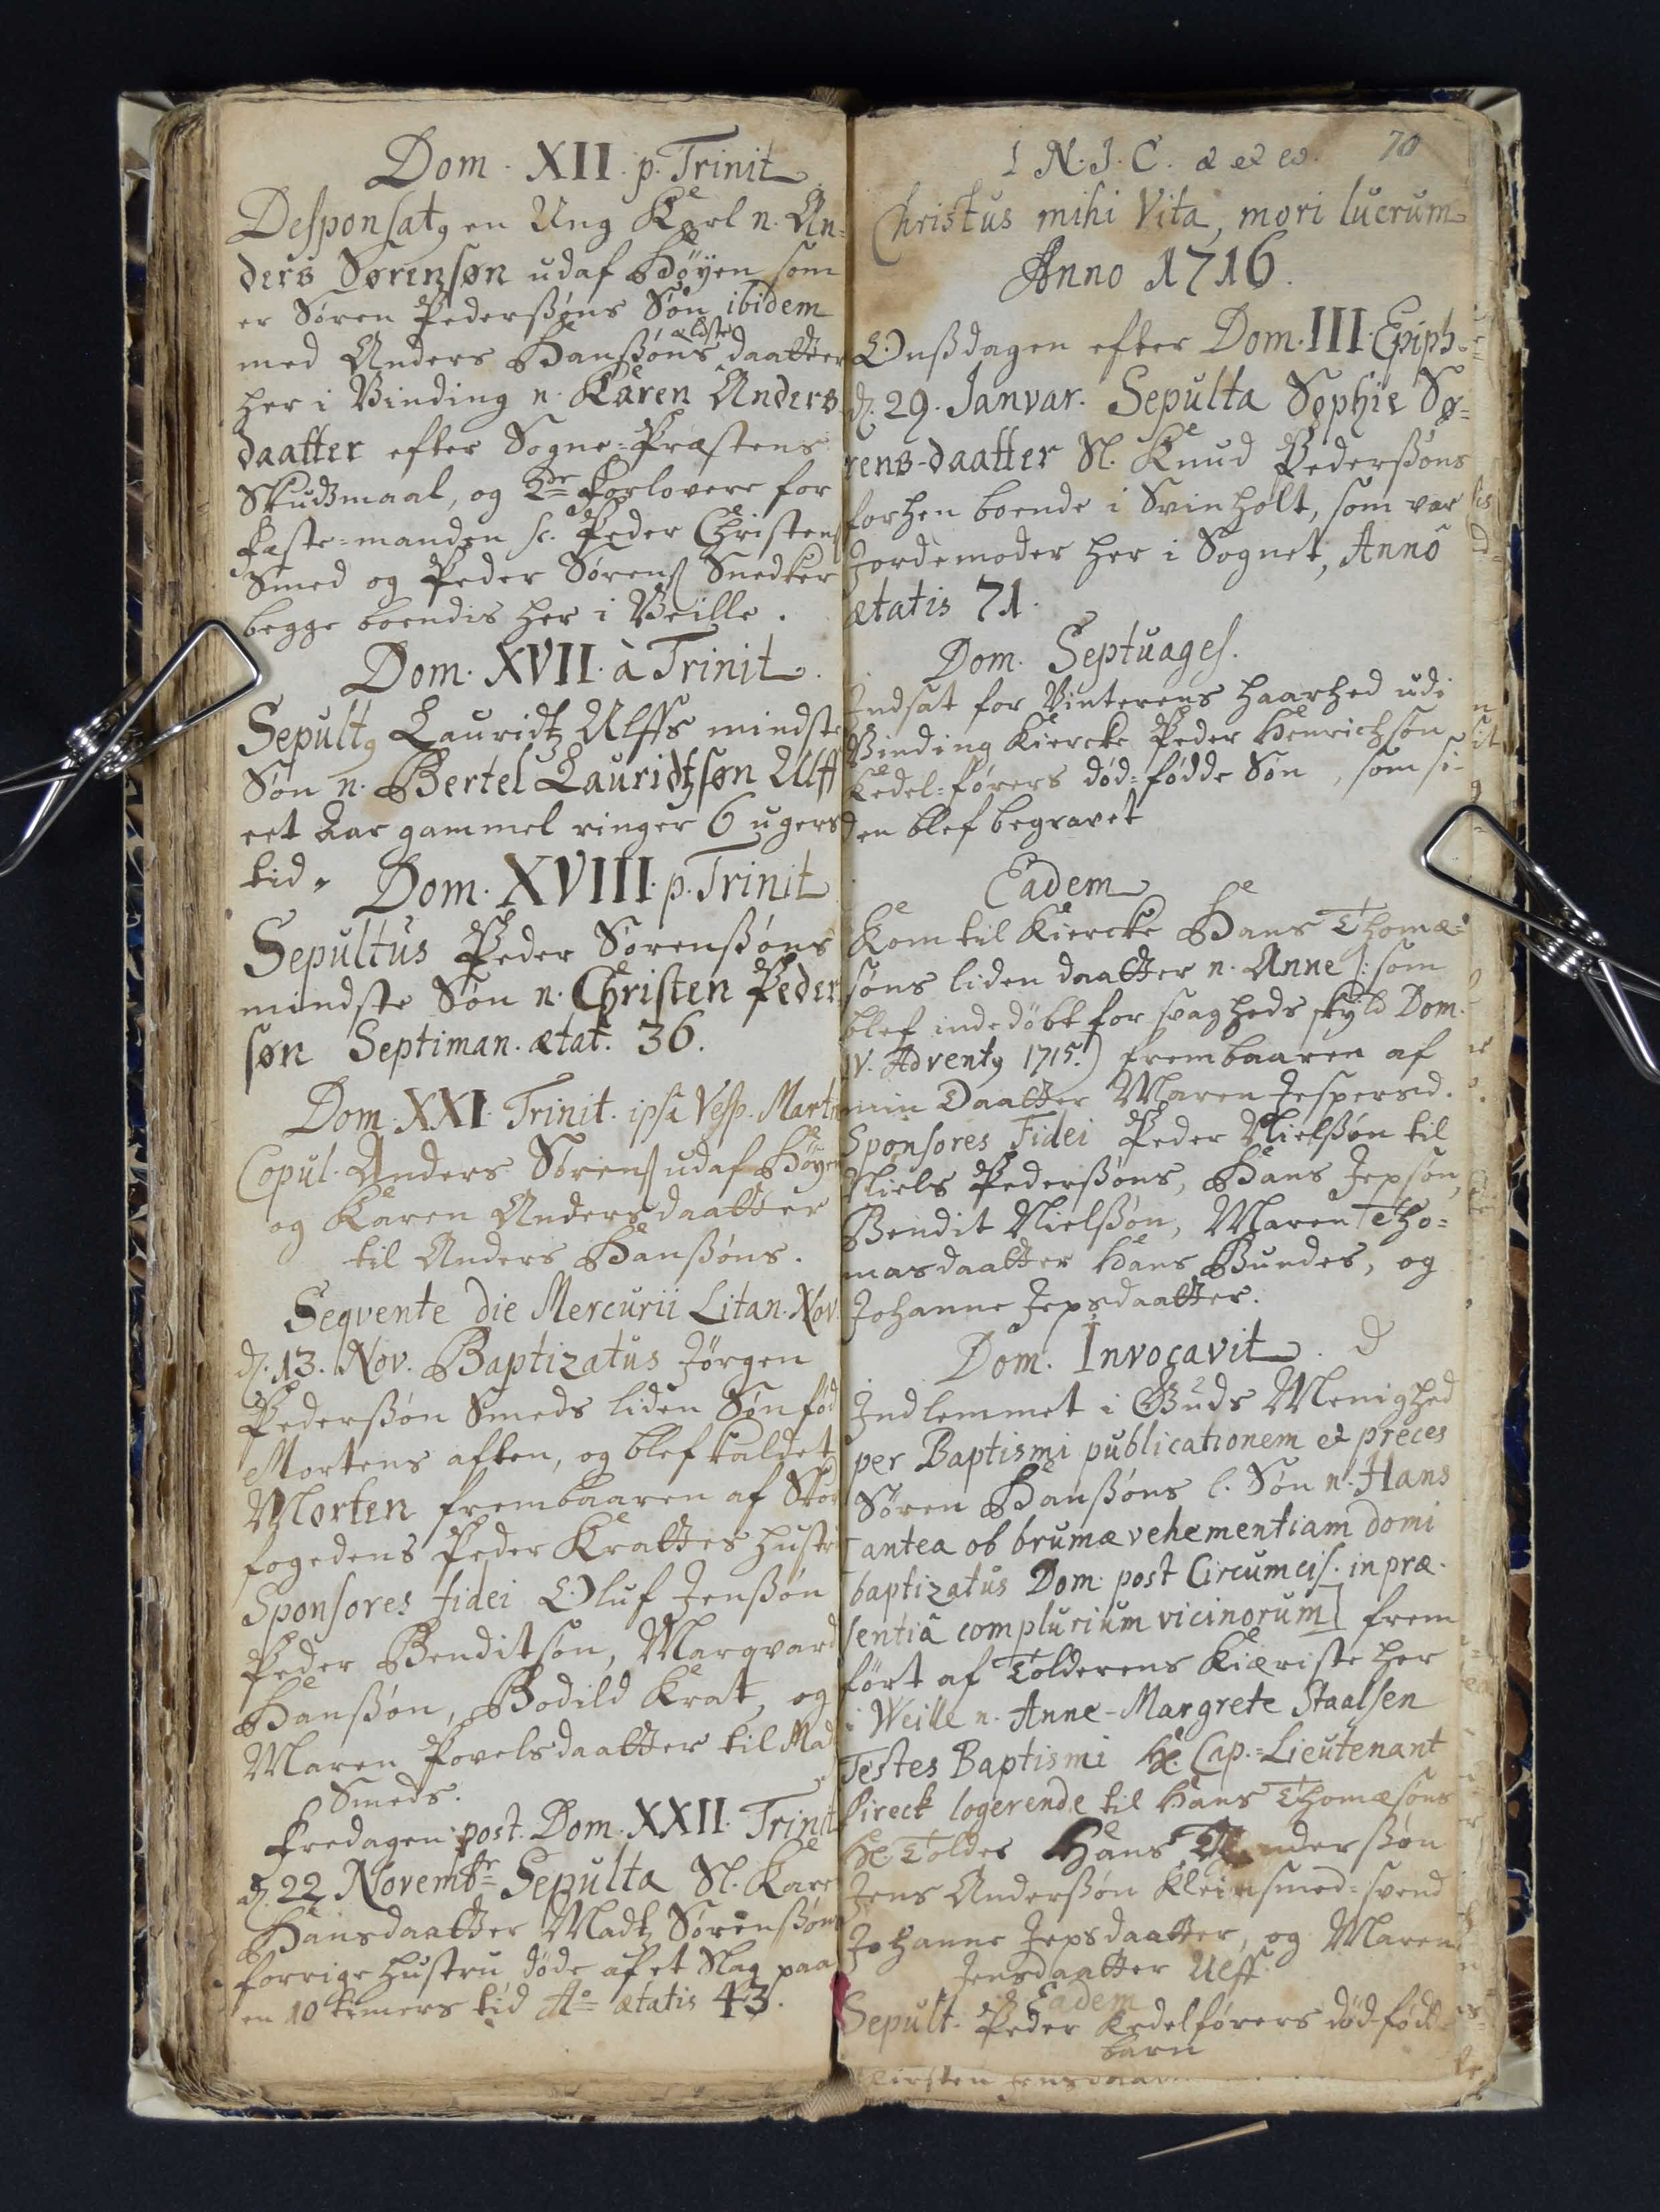Dom. XII. p. Trinit
Desponsatq en Ung Karl n. An
ders Sørensøn udaf Høyen som
er Søren Pederssøns Søn ibidem
ældste
med Anders Hanssøns daatter
her i Vinding n. Karen Anders
datter efter Sogne=Præstens 
Skudsmaal, og 2de Forlovere for
Fæste=manden sc. Peder Christens
Smed og Peder Sørens Snedker
begge boendis her i Veille.
Dom. XVII. a Trinit
Sepultq Lauridtz Ulffs mindste
Søn n. Bertel Lauridtzsøn Ulff
eet Aar gammel ringer 6 ugers
tid.
Dom. XVIII. p. Trinit
Sepultus Peder Sørenssøns
mindste Søn n. Christen Peder¬
søn Septiman. ætat. 36.
Dom. XXI Trinit. ipsa Vesap Martii
Copul. Anders Sørens udaf Høyen
og Karen Andersdaatter
til Anders Hanssøns.
Seqvente die Mercurii Litan. Nov.
d. 13. Nov. Baptizatus Jørgen
Pederssøn Smeds liden Søn fød
Mortens aften, og blef kaldet
Morten, frembaaren af Skov
fogedens Peder Krattis hustru 
Sponsores fidei Oluf Jenssøn
Peder Benditsøn, Margvard
Hanssøn, Bodild Krat, og
Maren Povelsdaatter til Mads 
Smeds.
Fredagen post Dom. XXII. Trinit
d. 22 Novembr Sepulta Sl. Karn 
Hansdaatter Madtz Sørenssøns
forrige hustru, døde af et Slag paa 
en 10 timers tid Ao ætatis 43.
70
I N. J. C. a et ##
Christus mihi Vita, mori lucrum
Anno 1716
Onssdagen efter Dom. III Epiph.
d. 29. Janvar. Sepulta Sophie Sø¬
rens-daatter Sl. Knud Pederssøns
forhen boende i Svinholt, som var 
Jordemoder her i Sognet, Anno 
ætatis 71
Dom. Septuages.
Indsat for Vinterens haarhed udi
Vinding Kiercke Peder Henrichsøn 
Kedel=førers død=fødde Som, som si¬
den blef begravet
Eadem
Kom til Kiercke Hans Thomæ¬
søns liden daatter n. Anne /: som
blef indedøbt for svagheds skyld Dom.
IV. Adventq 1715.) frembaaren af
min Daatter Maren Jespersd.
Sponsores Fidei Peder Nielsøn til
Niels Pederssøns, Hans Jepsøn,
Bendit Nielssøn, Maren Tho¬
masdaatter Hans Bundes, og
Johanne Jepsdaatter.
Dom. Invocavit
Indlemmet i Guds Menighed
per Baptismi publicationem et preces
Søren Hanssøns l. Søn n. Hans
[antea ob brumæ vehementiam domi
baptizatus Dom. post Circumcis. in præ¬
sentia complurium vicinorum] frem
ført af Tolderens Kiæriste her
i Weille n. Anne-Margrete Staalsen
Testes Baptismi Hl Cap.=Lieutenant
##ireck logerende til Hans Thomæsøns
Hl. Toldes Hans ##nderssøn
Jens Anderssøn Kleinsmed=svend
Johanne Jepsdaatter, og Maren
Jensdaatter Ulff.
Eadem
Sepult. Peder Kedelførers død-fødte 
barn
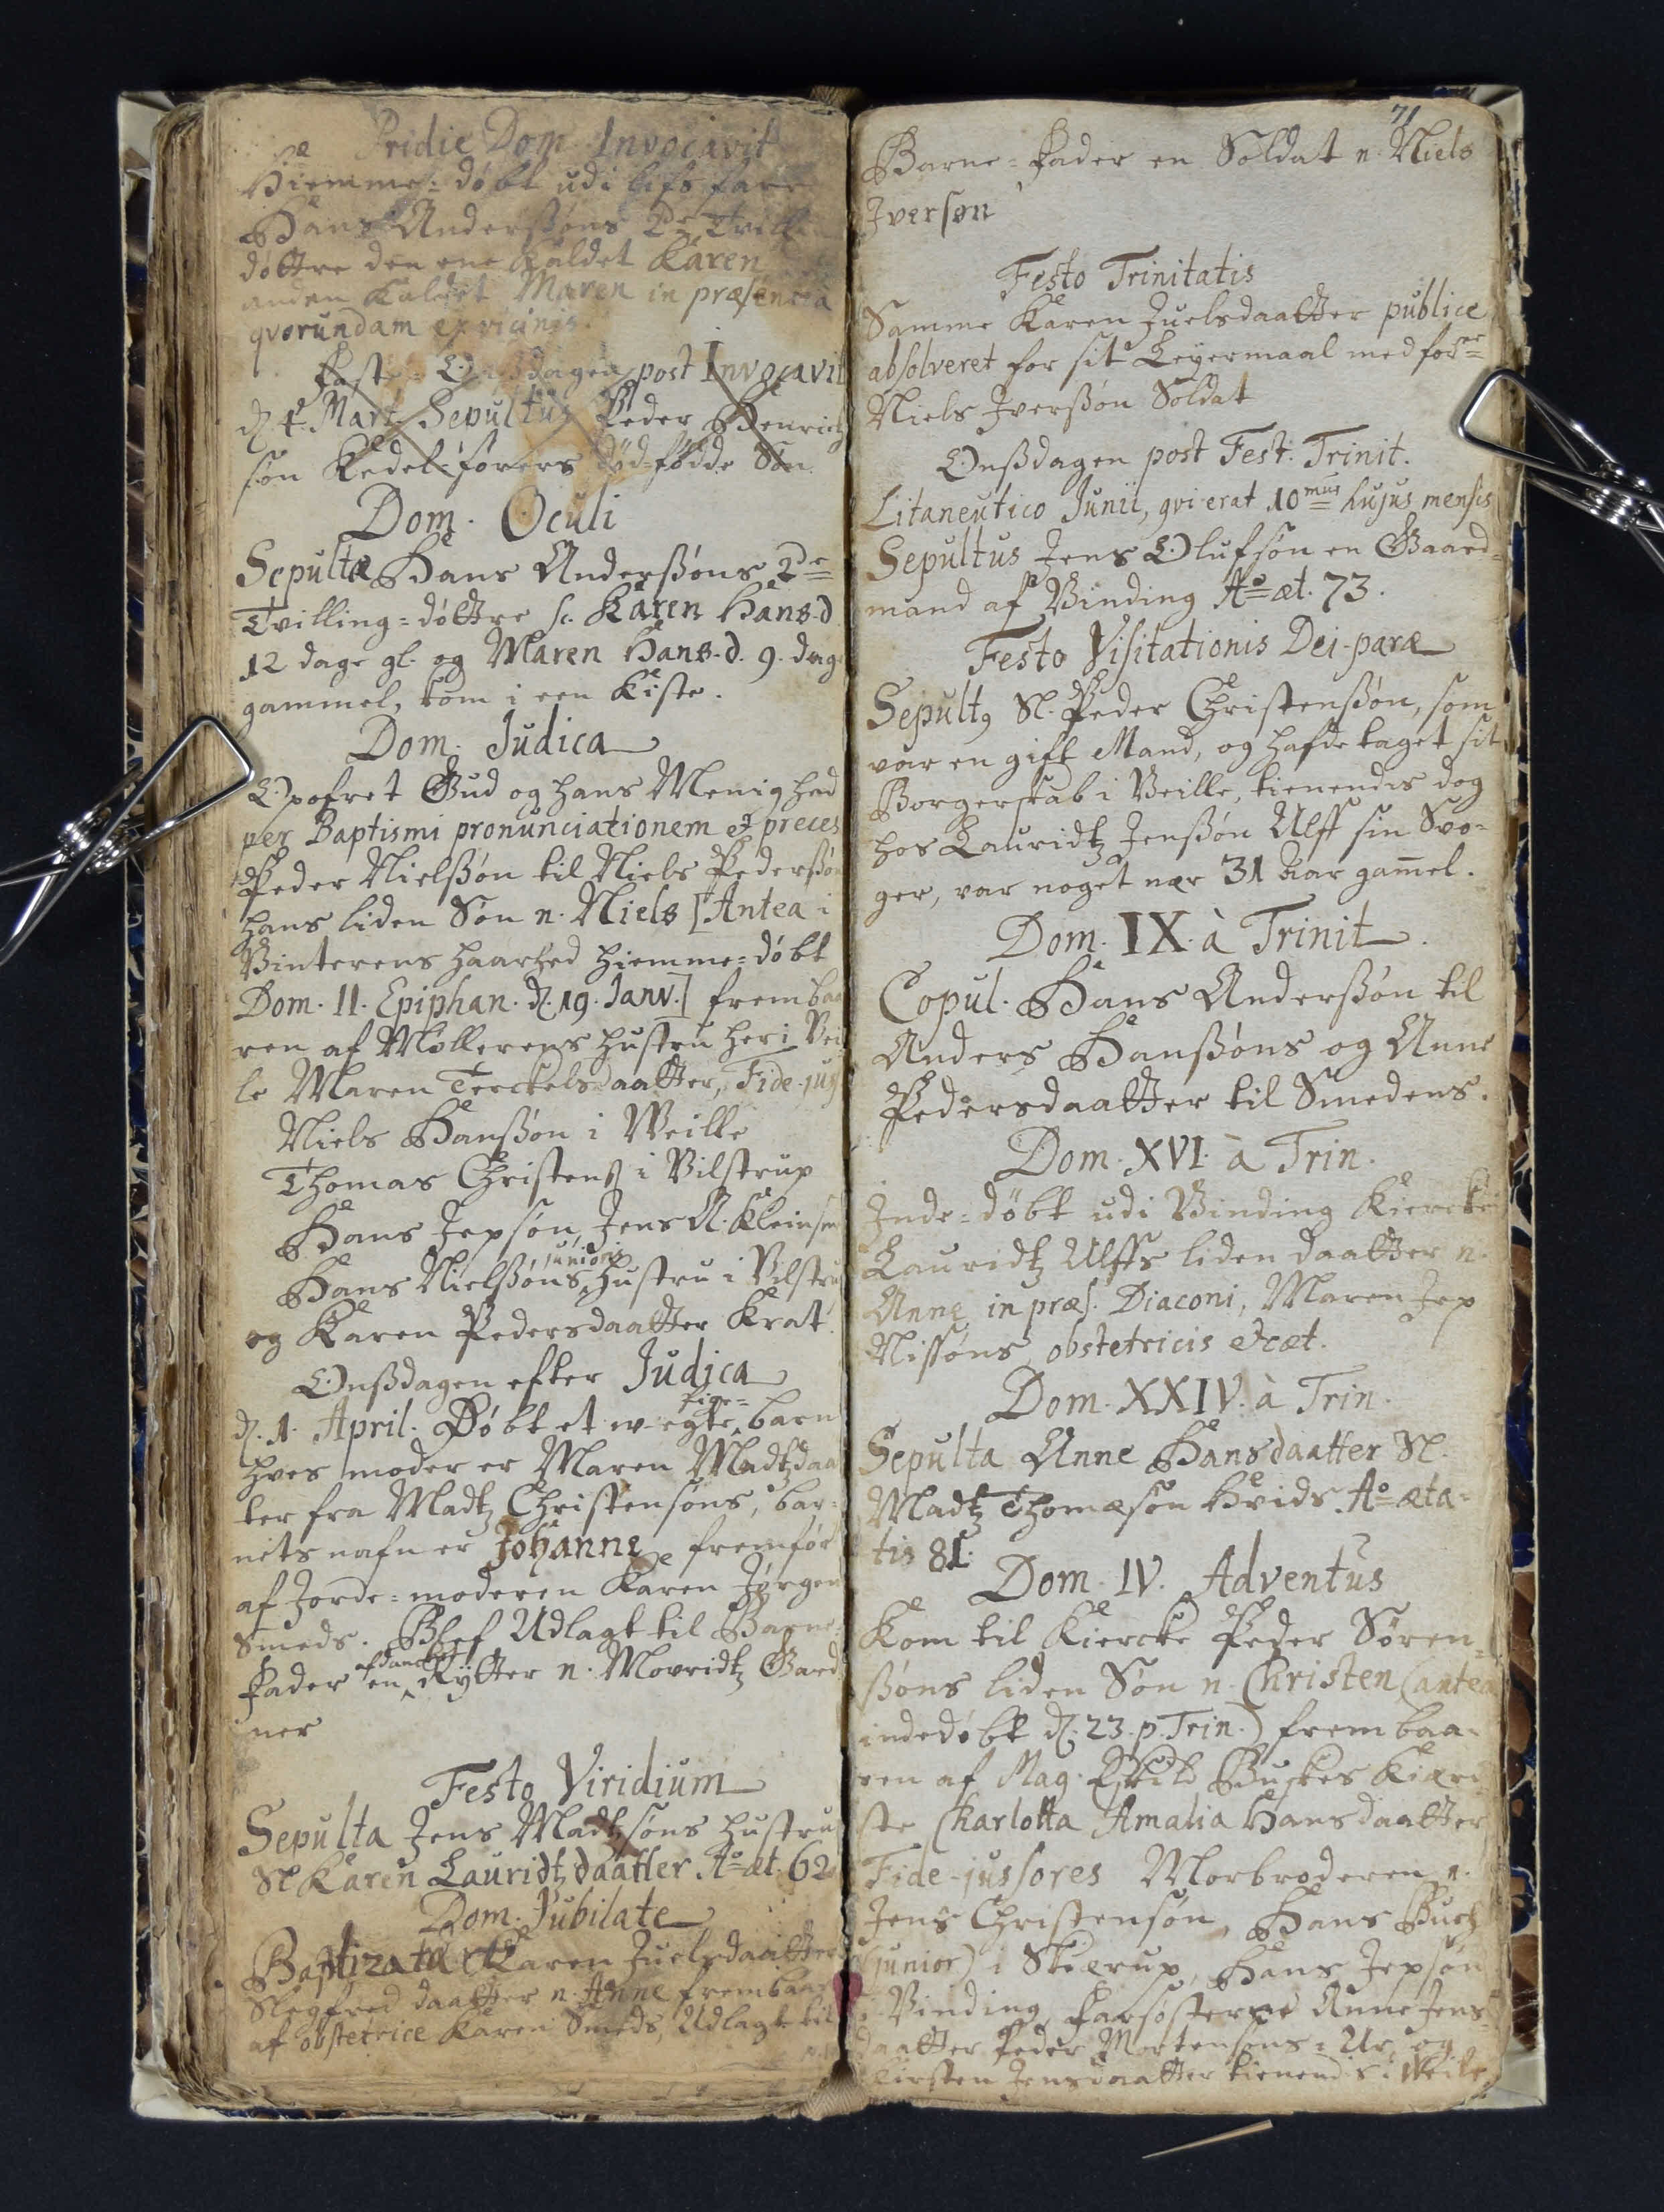Pridie Dom. Invocavit
Hiemme=døbt udi lifs fare
Hans Anderssøns 2de tvill##
døttre, den ene kaldet Karen ##
anden kaldet Maren in præsentia
qvorundam exvicinis.
Faste=Onssdagen post Invocavit
d. 4 Marts. Sepultus Peder Henrich 
søn Kedel-førers død=fødde Søn.
Dom. Oculi
Sepulta Hans Andesrsøns 2de
Tvilling=døttre sc. Karen Hans-d.
12 dage gl. og Maren Hans-d. 9 dage
gammel, kom i een Kiste
Dom. Judica
Opofret Gud og hans Menighed
per Baptismi pronuriciationem et preces 
Peder Nielssøn til Niels Pederssøn 
hans liden Søn n. Niels [Antea i
Vinterens haarhed hiemme=døbt
Dom. II. Epiphan. d. 19. Janv.] frembaa
ren af Møllerens hustru her i Vei¬
le Maren Terckelsdaatter, Fide-juss 
Niels Hanssøn i Weille
Thomas Christens i Vilstrup
Hans Jepsøn, Jens A. Kleinsm 
Juniors
Hans Nielssøn hustru i Vilstru 
og Karen Pedersdaatter Krat
Onssdagen efter Judica
Pige=
d. 1. April. Døbt et w-egte barn
hves moder er Maren Madtzdaa 
ter fra Madtz Christensøns, bar¬
nets nafn er Johanne fremfør 
af Jorde=moderen Karen Jørgen
Smeds. Blef Udlagt til Barne¬
afdancket
Fader en Rytter n. Movridtz Gard
ner
Festo Viridium
Sepulta Jens Madtzsøns hustru
Sl Karen Lauridtzdaatter Ao æt. 62
Dom. Jubilate
Baptizata Karen Juelsdaatter 
slegfred daatter n. Anne frembaar 
af abstetrice Karen Smeds, Udlagt til
71
Barne=Fader en Soldat n. Niels
Iversøn
Festo Trinitatis
Samme Karen Juelsdaatter publice
absolveret for sit Leyermaal med forne
Niels Iverssøn Soldat
Onssdagen post Fest. Trinit.
Litaneutico Junii, qvi erat 10mus hujus mensis
Sepultus Jens Olufsøn en Gaard¬
mand af Vinding Ao æt. 73.
Festo Visitationis Dei-paræ.
Sepultq Sl. Peder Christenssøn, som
var en gift Mand, og hafde taget sit 
Borgerskab i Veille, tienendes dog 
hos Lauridtz Jenssøn Ulff sin Svo¬
ger, var noget nær 31 Aar gammel.
Dom. IX. a Trinit
Copul. Hans Anderssøn til
Anders Hanssøns og Anne
Pedersdaatter til Smedens.
Dom. XVI. a Trin
Inde=døbt udi Vinding Kiercke
Lauridtz Ulffs liden daatter n.
Anne in præs. Diaconi, Maren Jep
Nissøns, obstetricis etcæt.
Dom. XXIV. a Trin.
Sepulta Anne Hansdaatter Sl.
Madtz Thomæsøn Hvids Ao æta¬
tis 81.
Dom. IV. Adventus
Kom til Kiercke Peder Søren¬
søns liden Søn n. Christen (antea
indedøbt d. 23. p. Trin.) frembaa¬
ren af Mag. Eskild Buskes Kiæri¬
ste Charlotta Amalia Hansdaatter
Fide-jussores Morbroderen n.
Jens Christensøn, Hans Buch
(junior) i Skiærup, Hans Jepsøn
i Vinding, Farsøsteren Anne Jens¬
daatter Peder Mortensøns i Ur, og
Kirsten Jensdaatter tienendis i Weile